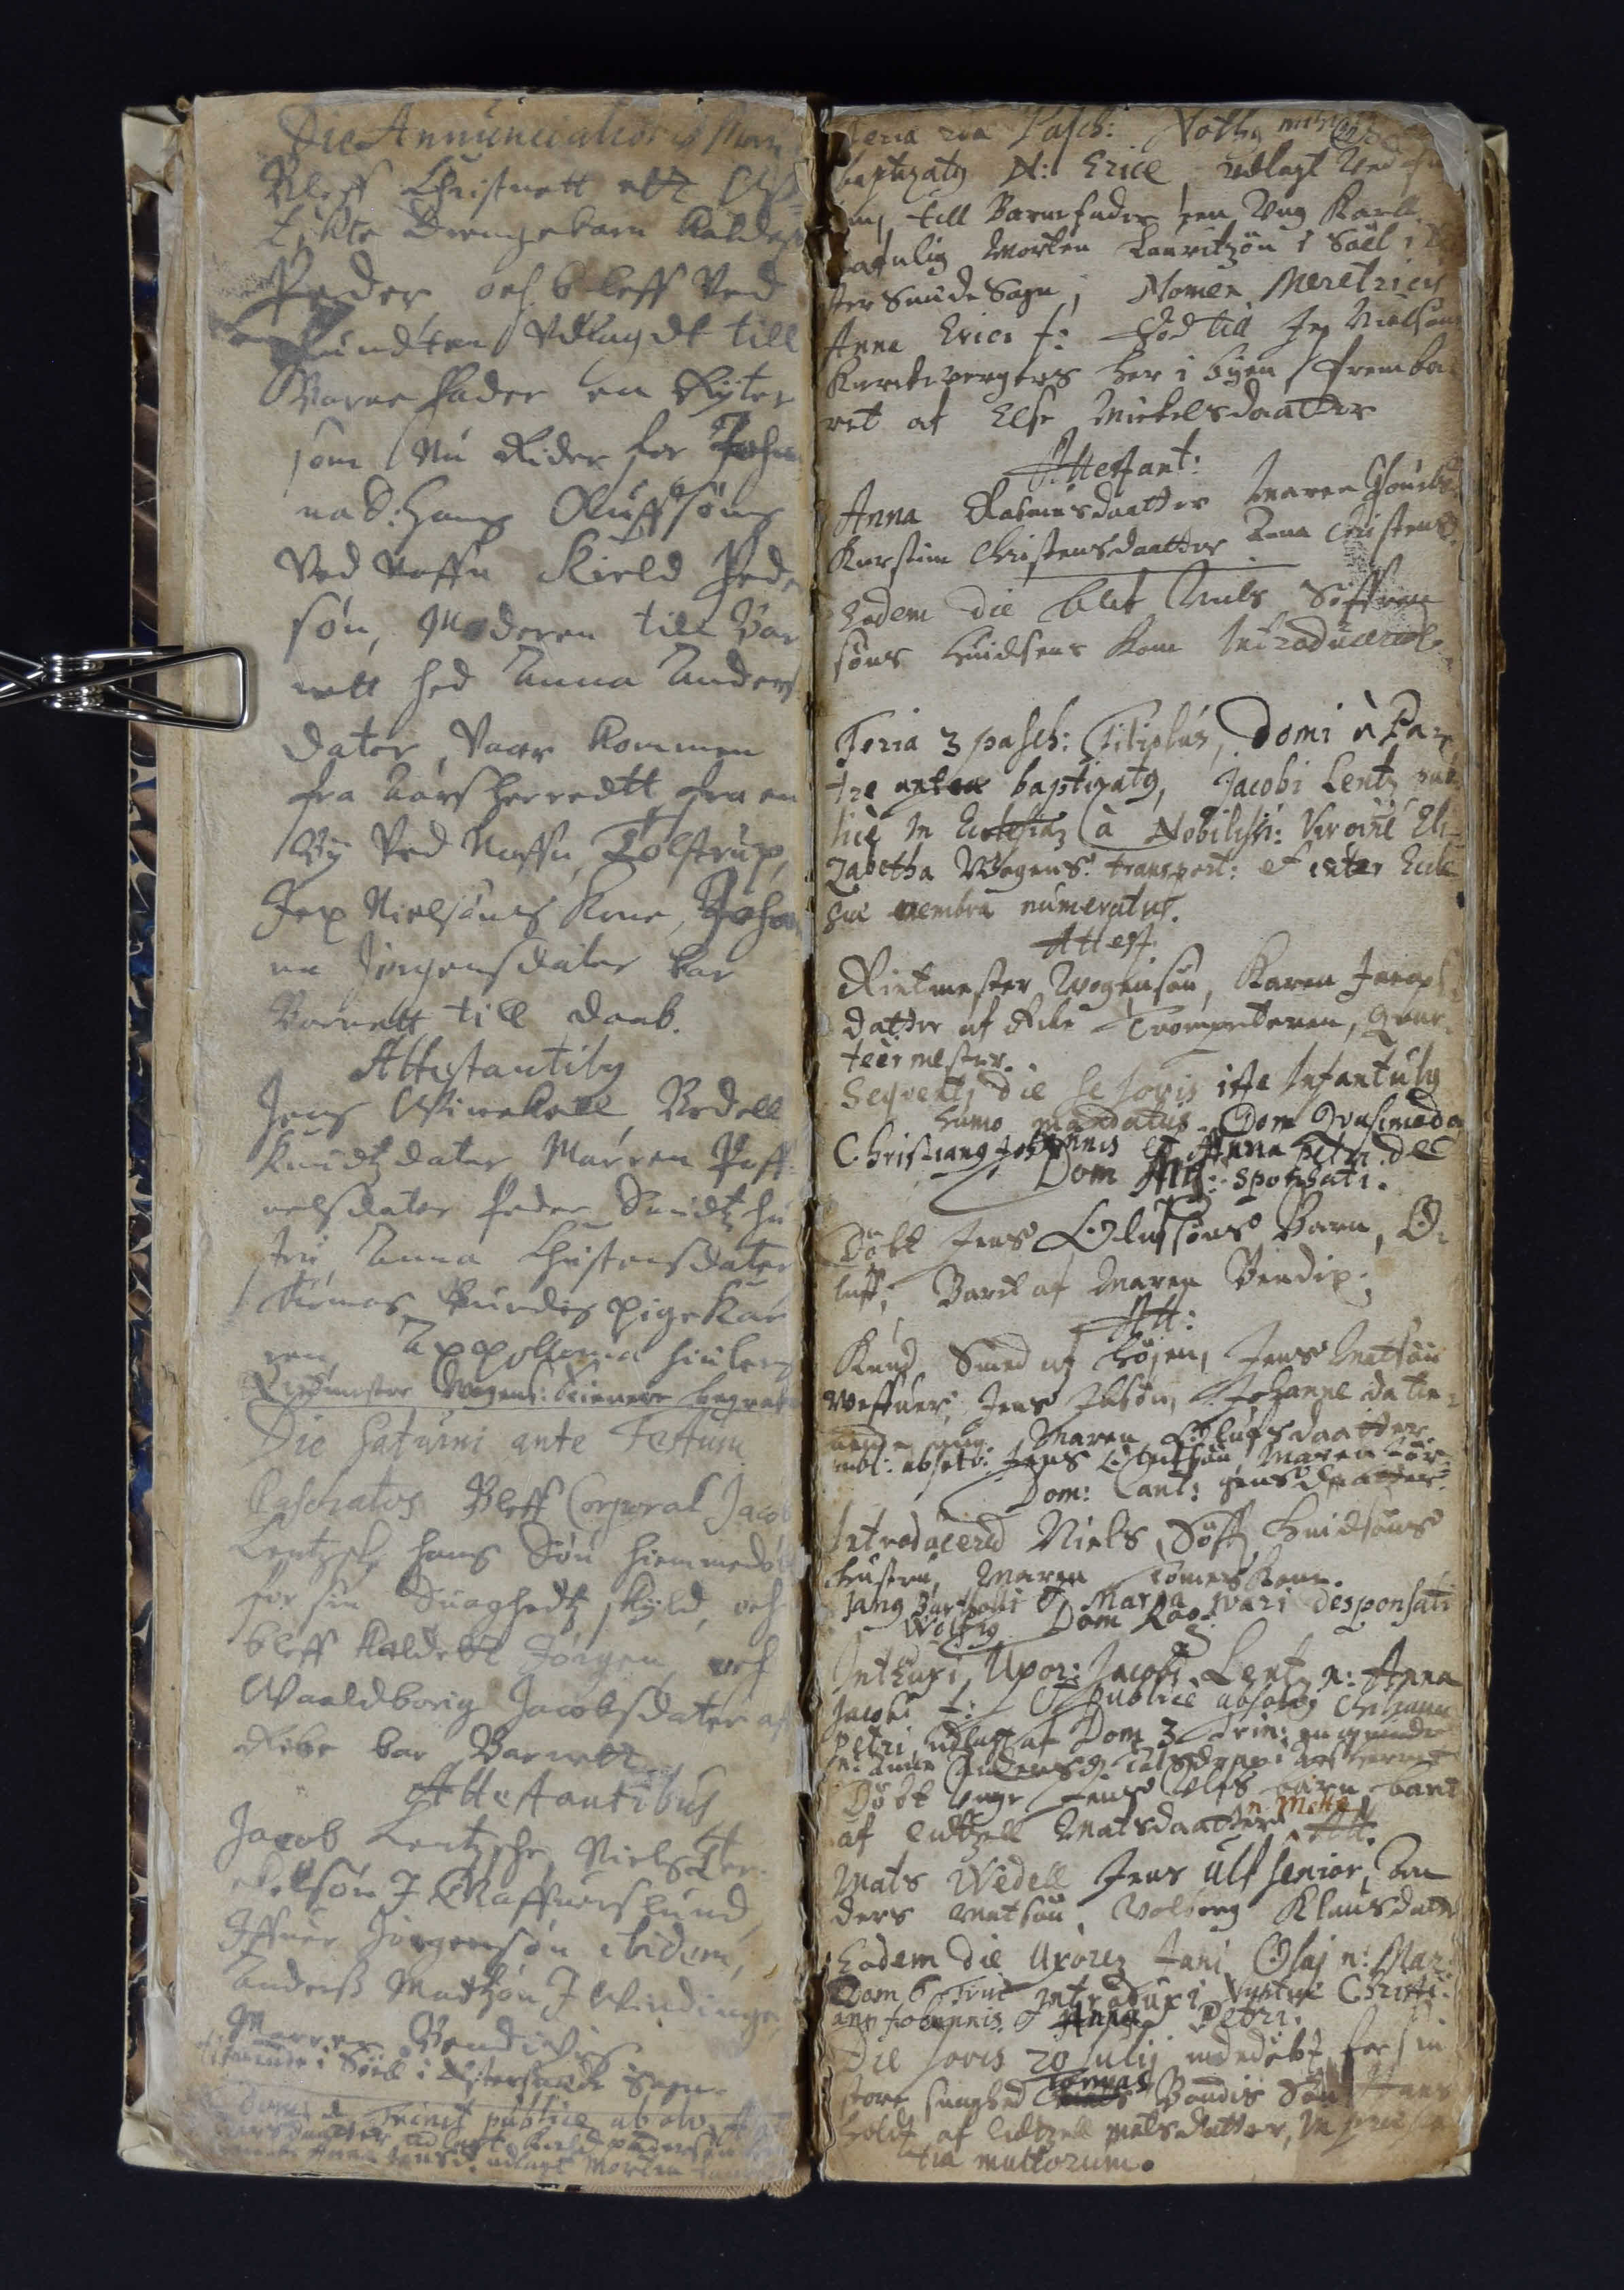Die Annuntiationis## Mar##
Bleff Christnet ett W¬
Eckte Drengebarn kaldet 
Peder, och bleff ved 
Fundten vdlagdt till
Barne Fader en Rytter
som nu Rider for Johan¬
na S: Hans Oluffsøns
Ved naffn Kield Peder
søn. Moderen till Bar
nett hed Anna Anders¬
dater, Vaar kommen
fra Aars herredtt fra en
By ved naffn Tolstrup,
Jep Nielsøns Kone Johan
na Jørgensdater bar
Barnett till daab.
Attestantiby
Jens Winckell. Bodell
Knudtzdater. Marren Poff¬
uelsdater. Peder Smidtz hu
stru Anna Christensdater
Thomas Bundis pige Kar
ren. Appollona Hiulers.
Ridtmester Wogens: tienner begraf 
Die Saturni ante Festum
Paschatos Bleff Corporal Jacob
Lentzshe hans Søn hiemmedø 
for sin suaghedtz skyld, och
bleff kaldett Jørgen, och
Waaldborig Jacobsdater af 
Ribe bar Barnett
Attestantibus
Jacob Lentzshe. Niels Ter¬
ckelsøn I Gaffuerslund
Iffuer Jørgensøn ibidem,
Anders Madtzøn I Windinge,
Marren Bendixis
i Siil i Østersnede Sogn.
Dnica. 1 Trinit. Publice ab##olv Anna
Andersdater udlagt Kield Pedersøn ##
## Anne Jensd. udlagt Morten Lauridtz 
22
Feria ##a Pasch: ##oth## mihi
baptizat## ##: Erice udlagt Ued fu 
till barnefader een Ung karl
afnlig Morten Lauridtzøn i Soel i
Snude Sogn; Nomen Meretricis
Anne Erice f##: ##ti## Jep Nielsøn
Kierckevergers her i byen frembor
ret af Else Michelsdaater
Attestant:
Anna Rasmusdaatter Maren Pouels
Kierstine Christensdaatter Anna
Eodem die. blef Niels Søffren
søns Hvidsens Kone Introduceret.
Feria 3 Pasch: Filiolus, Domi a Pa¬
tre antea baptizat##, Jacob Lentz pub
lice In Antesia a Nobilise:. Viroire Eli¬
zabetha Wogens transport. ef## ## Eccle
sia membra numeratus. 
Attest:
Ritmester Wogensøn. Karen Jacob 
datter af Ribe. Trompeteren Qvar
teermester.
Seqvetti die sc Jovis 1ste Infantu
humo mandatus Dom. Qvasimodo
Johannis et Anna Petri De
Dom A##: sponsati.
Døbt Jens Olufsøns Barn O¬
luff; Baaret af Maren Bendix
Att:
Knud Smed af Højen, Jens Matsøn
Weffuer; Jens Ibsøn, Johanne da tie¬
enende mig. Maren Olufsdaatter.
absolv: Jens Olufsøn, Maren Jør¬
gensdaater.
Dom: Cant:
Introducered Niels Søff Huidsøns
hustru Maren Thomas Kone.
Dom. Rog.
Jan## Bartholli et Mar##a Ivari desponsati
Int## Upor##: Jacobi Lentz ##: Anna
Jacobs ##: et publice absolv## Christjann##
Petri udlagt af Dom 3 Trin: en qvinde
n. Anna Andersd. Tolsdorp i Aars Herrit
Døbt Unge Jens Ulfs barn 
n. Mette
baret
af Cidtzell Madtzdaatter.
Att. 
Mats Wedell. Jens Ulf senior, An
ders Matsøn, Volborg Klausdatter
Eodem die Uxoriz Jani Olaj n. Mar:
Dom 6 trin Introduxi. Nuptia Christi¬
ane Johannis Anna Petri.
Die sovis 20 Julij. indedøbt for sin
Tomas
store svaghed ## Bundis Søn Hans
holdt af Cidtzell Matsdatter, in præsen
tia Muttorum.
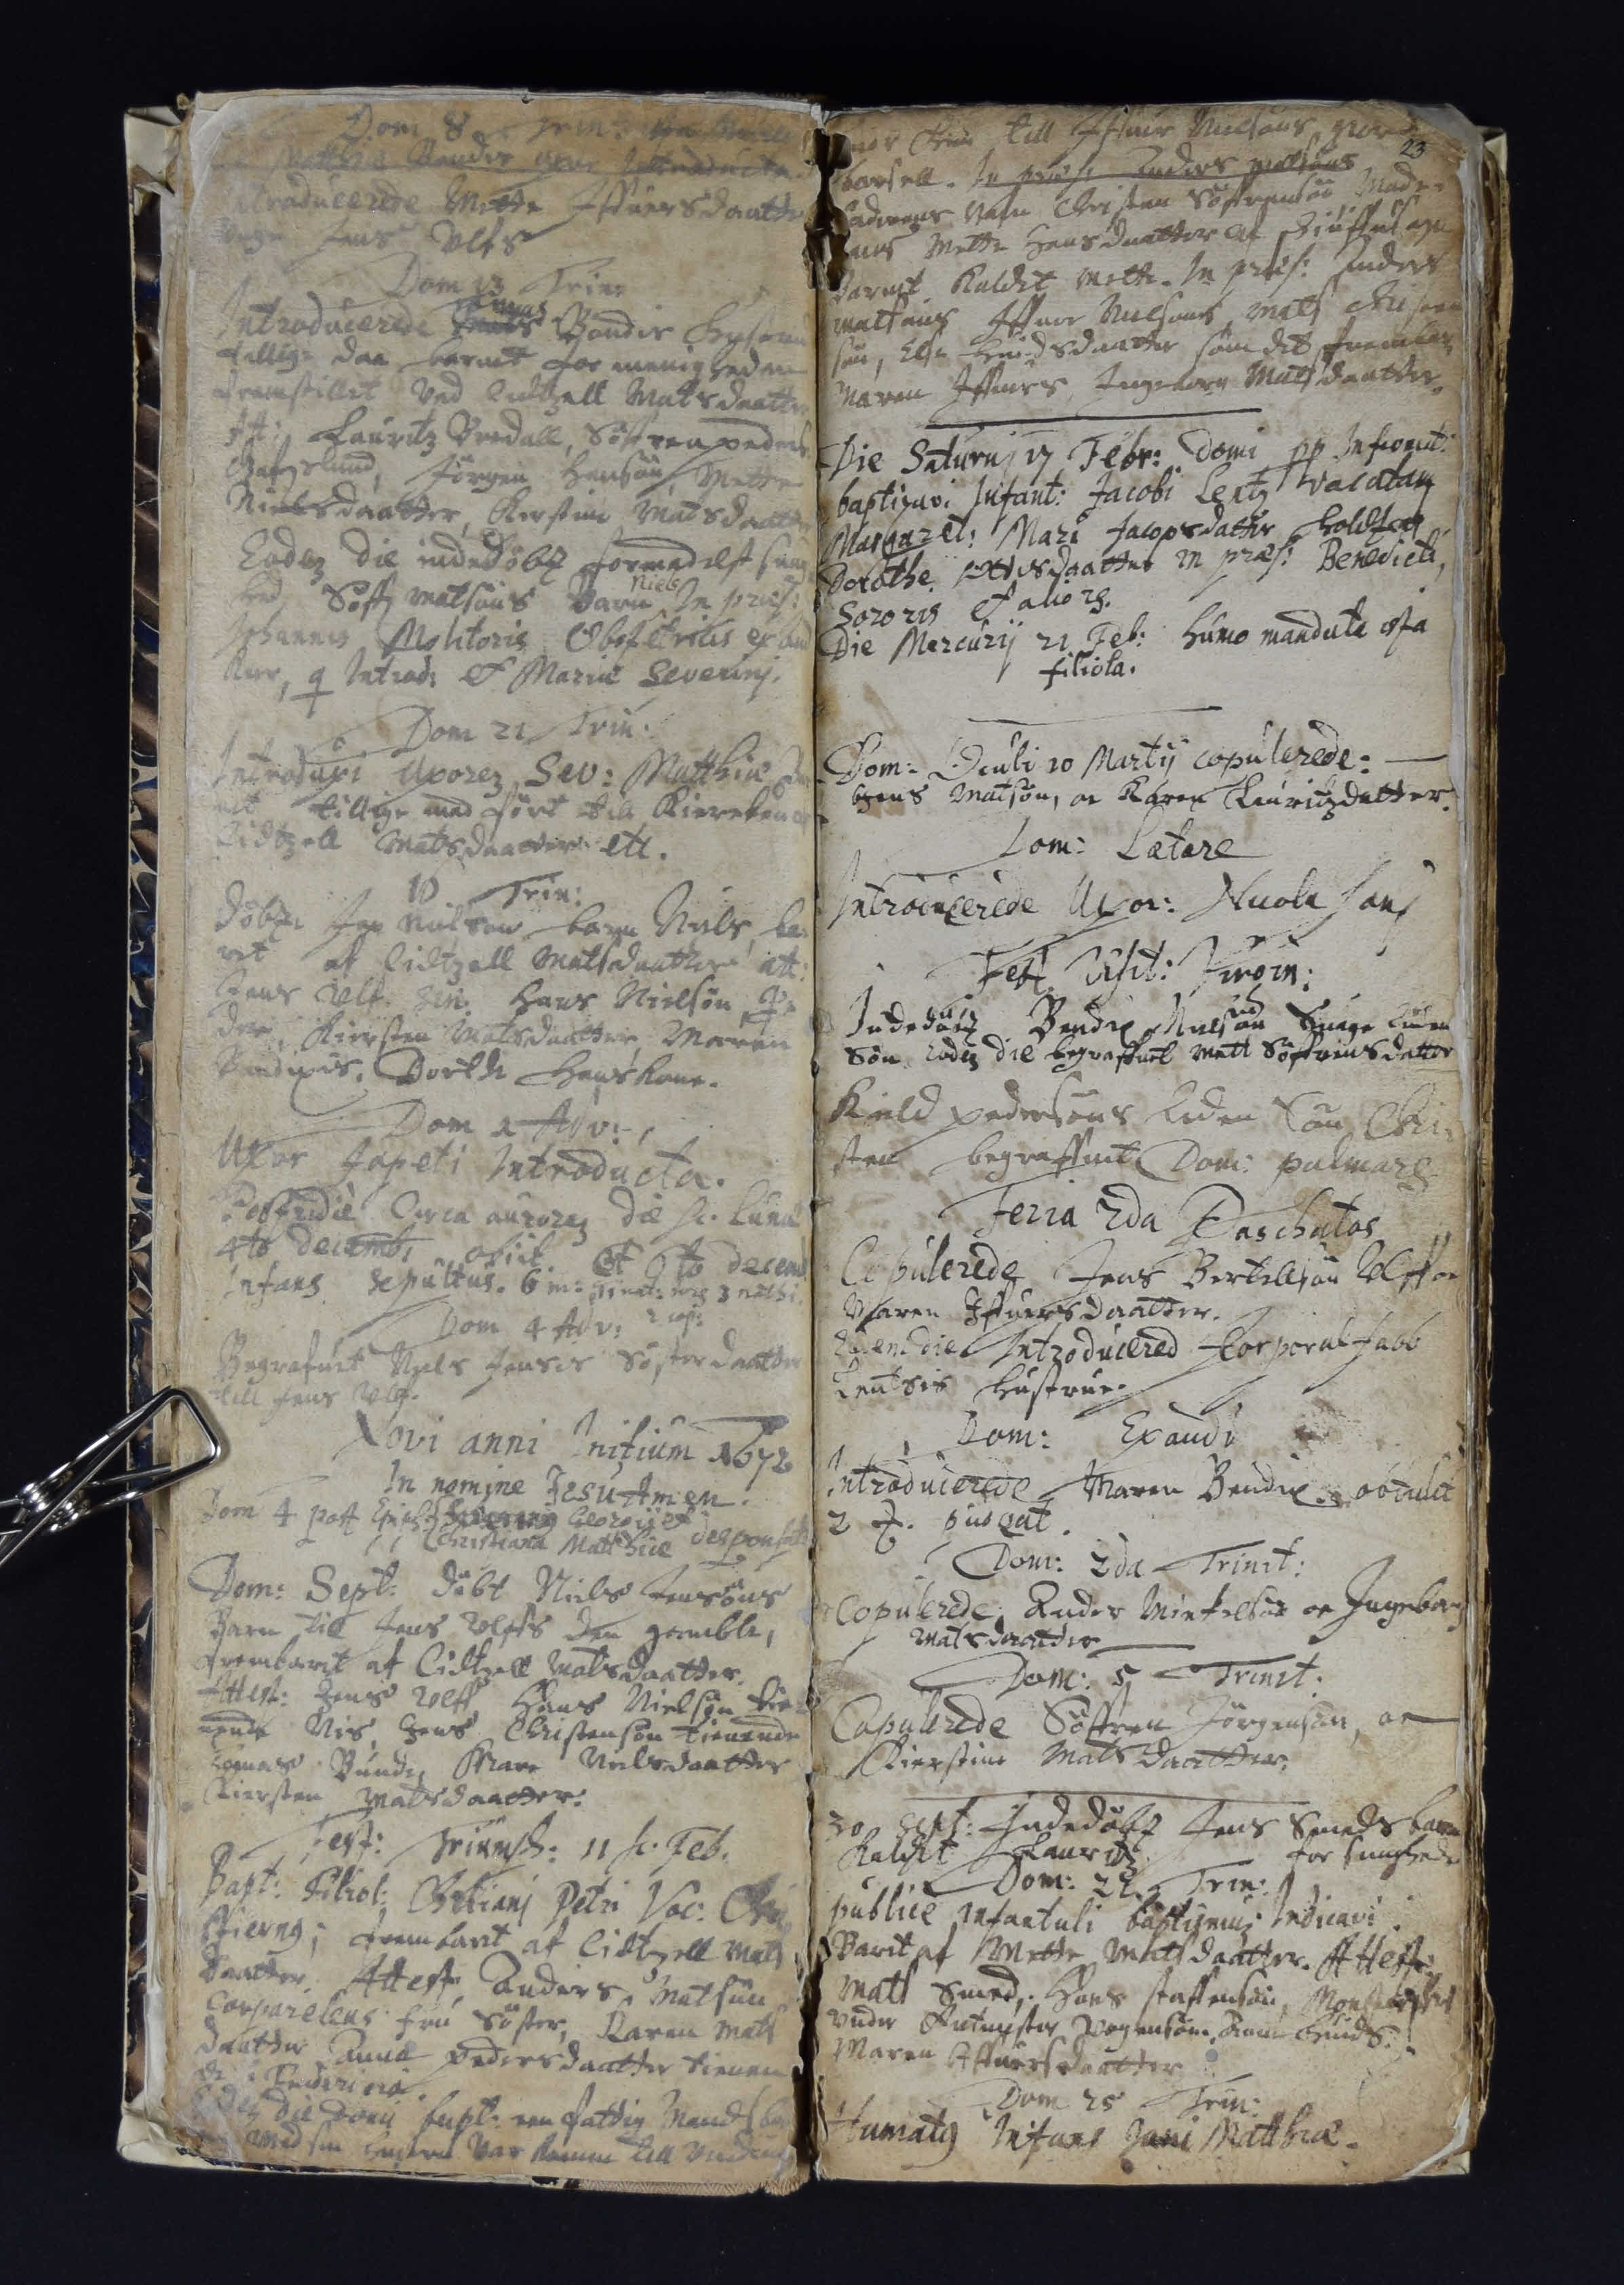Dom. 8 p Trin: ## ##
## Matthis Bundes ## Introducta
Mette Iffuersdaatter
unge Jens Vlfs
Dom. 13 Trinit.:
Thomas
Introducerede ## Bondis hustru
tillige daa barnet for menigheden
fremstillet ved Cidtzell Matsdaatter
Att: Lauritz Bredall. Søffren Peders.
Nielsdaatter, Kirstine Matsdaatter
Jørgen Hansøn. Mette
Eodem die indedøbt formedelst suag
Niels
hed Søff Matsøns Barn In præs:
Johannes Mohtoris Obefetrius## ex And
kier, q Introd: et Maria Severinj.
Dom. 21 Trin
Introduxi Uxorez Sev: Matthia Bar
net tillige med ført till Kiercken af
Zidtzell Matsdaatter. etc.
16. Trin:
Døbt Jep Nielsøns barn Niels ba¬
ret af Cidtzell Matsdaatter att:
Jens Vlf zen: Hans Nielsøn Pe¬
der, Kiersten Matsdaatter, Marren
Bendixis. Dorthe Hans Kone
Dom. 1 Adv:
Uxor. Japeti Introducta
##die ##ca aurorez dæ sc. Lunæ
4to Decemb, obiit. et 6to Decemb.
Infans sepultus 6 m. 11 nat: ##g 3 nathi.
2 cop:
Dom. 4 Adv:
Begrafuet Niels Jensis Søsterdaatter
till Jens Vlf.
Xovi anni Initium 1672
In nomine Jesu Amen
Dom 4 post Epiph Severin## Georgij et
Christiana Matthiie desponset##
Dom: Sept: døbt Niels Jensøns
Barn til Jens Vlfs den gamble,
frembarit af Cidtzell Matzdaatter.
Attest: Jens Ulff Hans Nielsøn tie¬
nende Nis, Jens Christensøn tienende
Thomas Bunde, K##ar## Nielsdaatter.
Kiersten Matsdaatter.
Fest: Tri##: 11 sc. Feb.
Bapt: Filiol: Chritianj Petri Voe. Chri
stiern##, frembart af Cidtzell Mats
daatter. Attest, Anders Matsøn.
Corporielens fru Søster, Karen Mats
daatter Anna Pedersdaatter tienen
de i Fridericia.
Die Domi bapt: en fattig mands ba 
med sin hustru var komen till Vindin 
23
## Chri## till Iffuer Nielsøns giorde##
Barsell. In ## Anders ##
faderens Nafn Christen Søfrensøn. Moder¬
ens Mette Hansdaatter af Schiuffu sogn
Barnet kaldtz Mette. In pr##is: Anders
Matsøns Iffuer Nielsøns, Mats Christen
søn. Else Hvidsdaatter som det frem bar,
Maren Iffuers, Ingeborg Matsdaatter
Die Saturuj 17 Febr; Domi pp In fioant:
baptizavi Infant:. Jacobi Lertz
Margeret: Mari Jacopsdatter, 
Dorothe Ottisdaatter in præs: Benedicti
sororis et alio
Die Marcurij 21 Feb: Humo mandate
filiola.
Dom. Oculi. 20 Martij Copulerede:
Hens Matsøn, oc Karen Lauritzdatter.
Dom: Lætare
Introducerede Uxor Nicola Jani.
Fest Usit:## ##
Indedøbt Bendix Nielsøns Suage
Søn. Eode## Die begrafuet Mett Søffrensdatter.
Kield Pedersøns liden Søn Chri
sten begrafuet Dom: Palmare
Feria 2da Paschatos
Copulerede Jens Bertelsøn 
Iffuersdaatter.
Eodem Die. Introducered Corporal Jacob
Lentsis Hustrue.
Dom: Exaudi.
Introducerde Maren Bendix, obtulit
2 mk.
Dom. 2da Trinit:
Copulerede Anders Mickelsøn oc Ingeborg
Matsdaatter
Dom: 5 Trinit:
Copulerede Søffren Jørgensøn oc
Kierstine Matsdaatter
30 Sept. Indedøbt Jens Smeds barn
kaldet Lauritz for
Dom: 22 Trin:
Publice infantuli baptisnius Indicavi 
Baret af Mette Matsdaatter. Attestr
Mats Smed,. Hans Staffensøn, Morten
under Ritmester Wogensøn. Anna Huids.
Maren Iffuersdaatter
Dom 25 Trin:
Humatus Infans Jani Matthia.